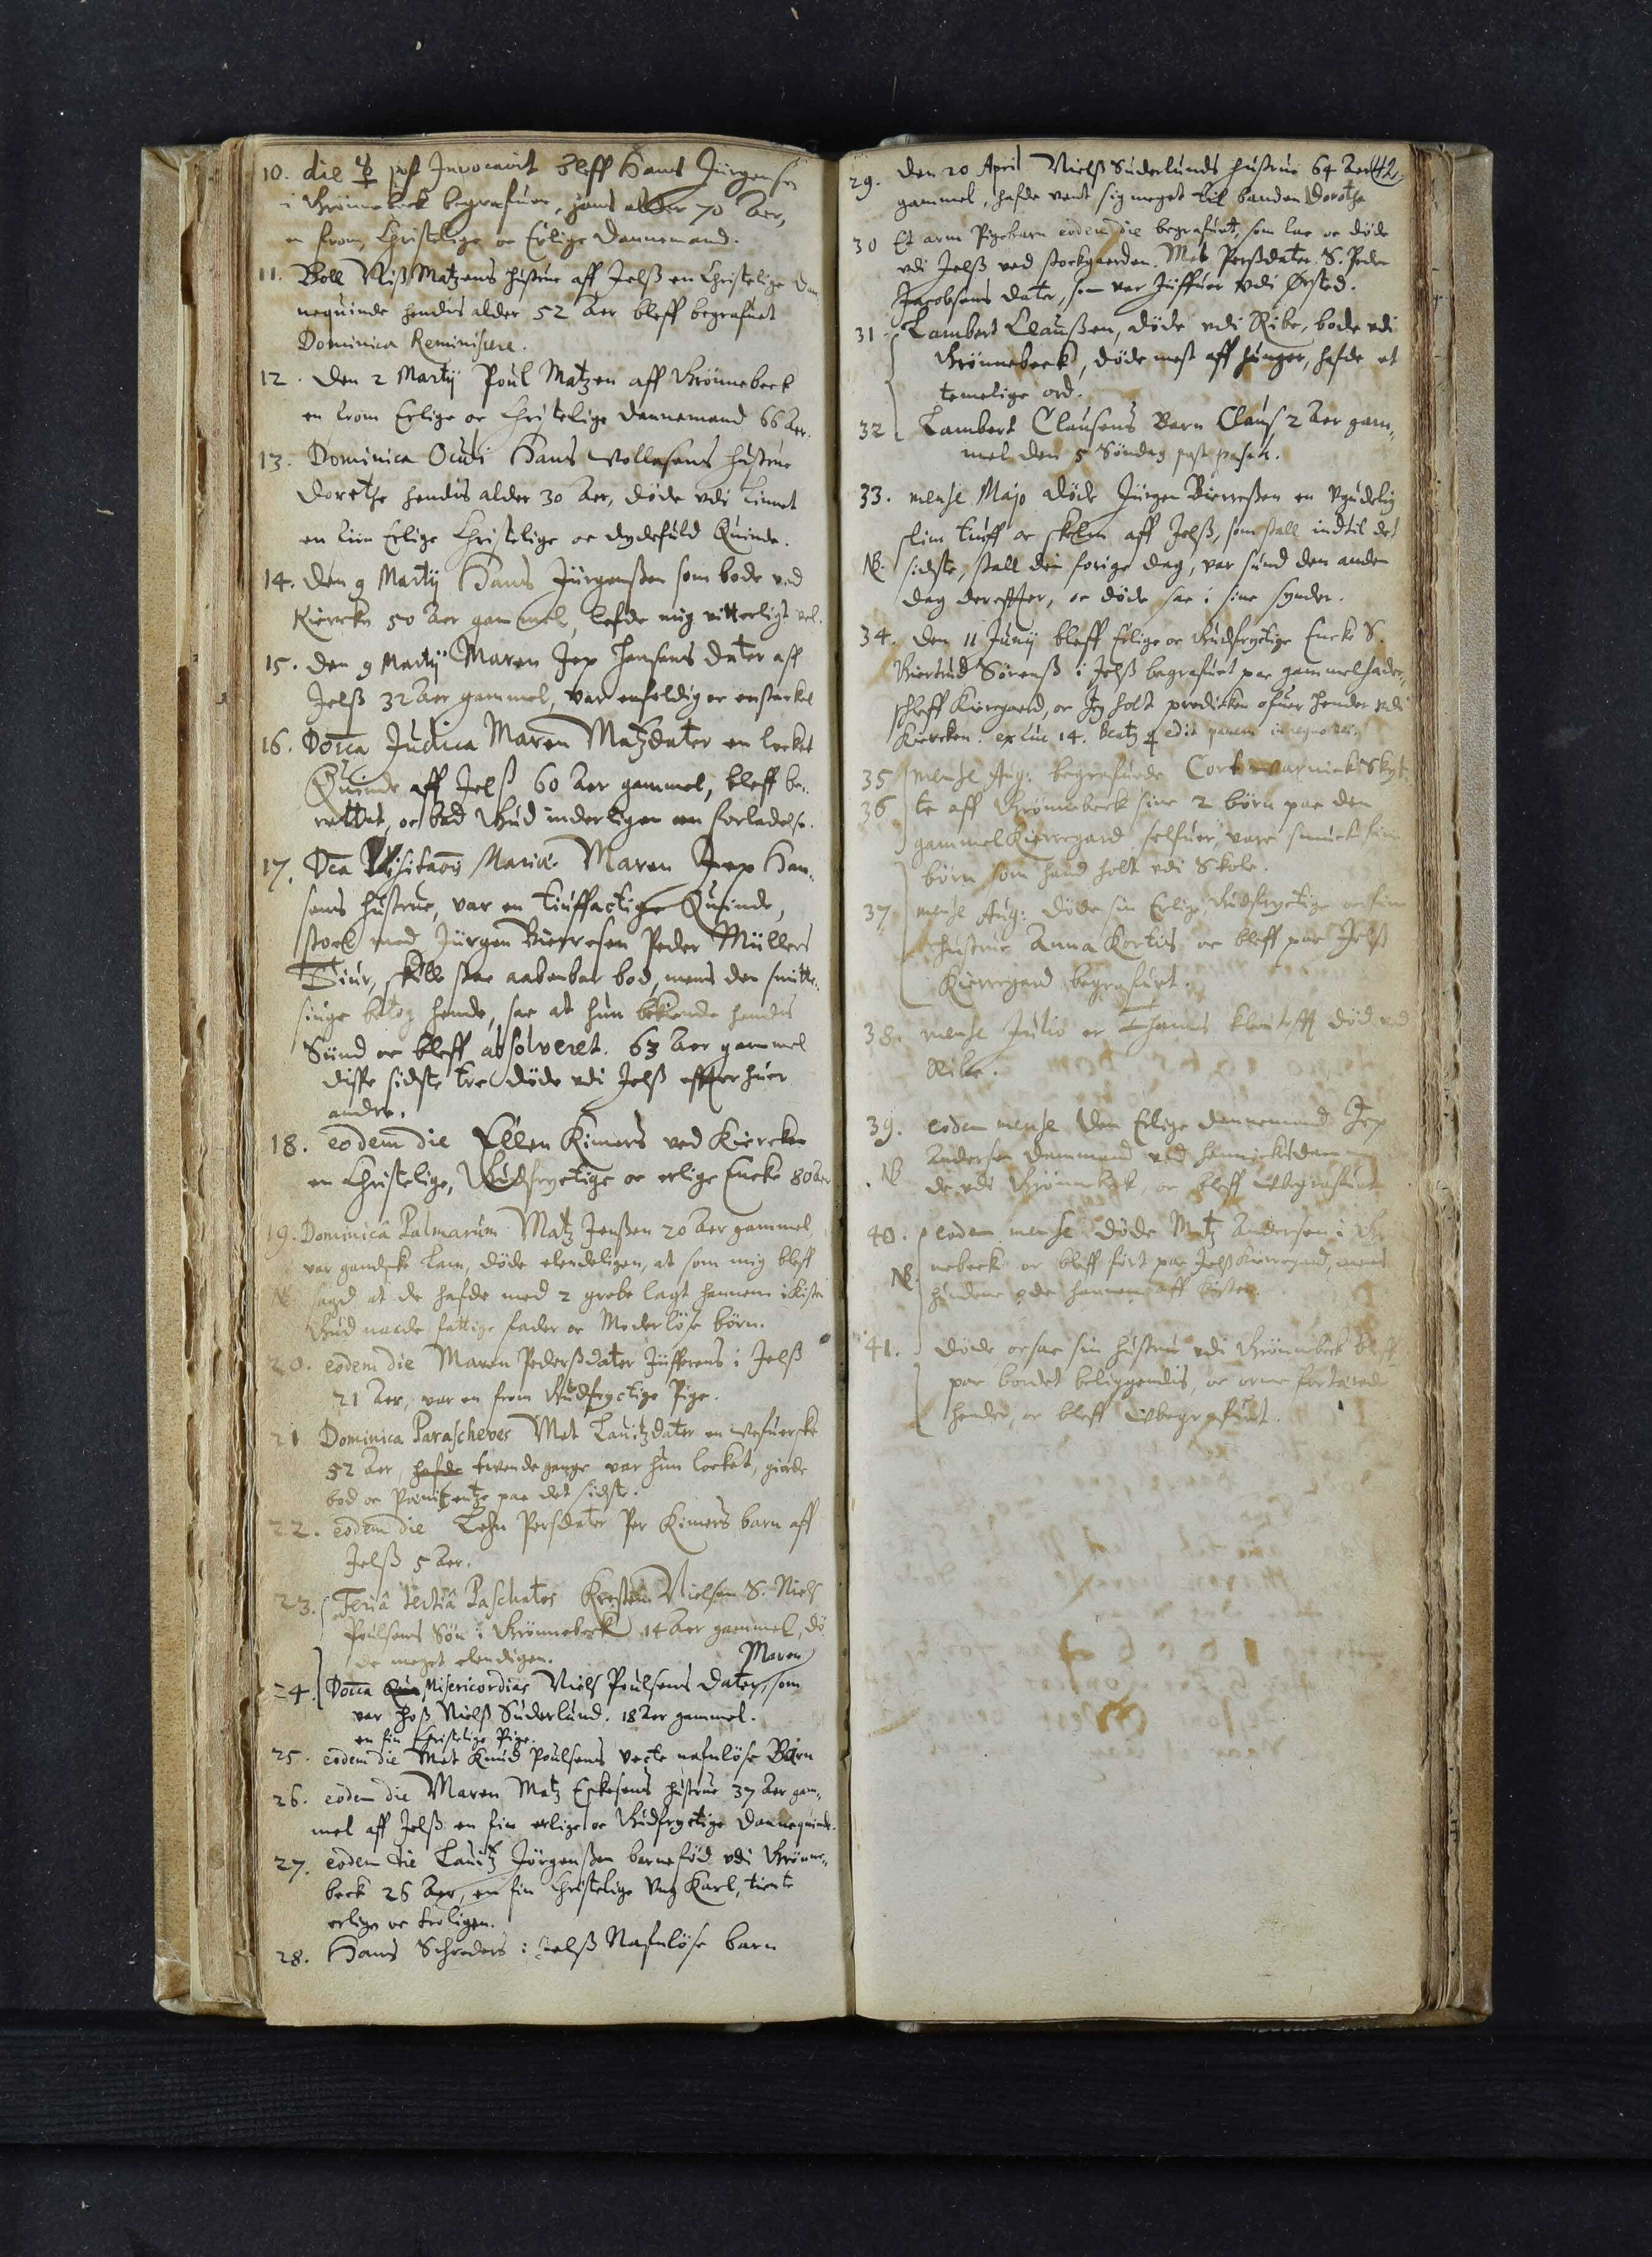10. die ☿ 8 post Invocavit bleff Hans Jurgensen
i Grønnebeck begrafuet, hans alder 70 Aar,
en from Christelige oc Erlige Dannemand.
11. Boll Niss Matzens hustrue aff Jelss en Christelige Dan¬
nequinde hendis alder 52 Aar bleff begrafuet 
Dominica Reminiscere.
12. Den 2 Martijy Poul Matzen aff Grønnebeck
en from Erlige oc Christelige Dannemand 66 Aar.
13. Dominica Oculi Hans Vollesens hustrue
Dorethe hendis alder 30 Aar, døde vdi Linnet
en fiin Erlige Christelige oc dydefuld Quinde.
14. Den 9 Martij Hans Jurgensen som bode ved
Kiercken 50 Aar gammel, lefde mig vitterligt vel.
15. Den 9 Martij Maren Jep Hansens Dater aff
Jelss 32 Aar gammel, var enfoldig oc en stackel.
16. Doica Judica Maren Matzdater en locket
Quinde aff Jels 60 Aar gammel, bleff be¬
rettet, oc bad Gud inderligen om forladelse.
17. Dca Visitaonis Maria. Maren Jep Han¬
sens hustrue, var en tiuffactige Quinde,
stoel med Jurgen Bierresen Peder Mullers
Tiuv, skulle stae aabenbar bod, men den smitte¬
siuge betog hende, sae at hun bekiende hendis
Sund oc bleff absolveret. 63 Aar gammel
Disse sidste tre døde vdi Jelss efter huer
andre.
18. eodem die Ellen Kimers ved Kiercken
en Christelige, Gudfryctige oc erlige Encke 80 Aar
19. Dominica Palarum Matz Jenssen 20 Aar gammel,
var gandske lam, døde elendigen, at som mig bleff
NB sagd at de hafde med 2 grebe lagt hannem i kisten
Gud naede fattige fader oc Moderløse børn.
20. eodem die Maren Pederssdater Jufferens i Jelss
21 Aar, var en from Gudfryctige Pige.
21. Dominica Parascheves Met Lauitzdater en vefuerske
52 Aar, hafde tvende gange var hun locket, giorde
bod oc Psnitentze paa det sidste.
22. eodem die Lehn Persdater Per Kimers barn aff
Jelss 5 Aar.
23. Feria tertia Paschatos Kresten Nielsen S. Niels
Poulsens Søn i Grønnebeck 14 Aar gammel, dø
de meget elendigen.
Maren
24. Docca ## Misericordias Niels Poulsens Dater, som
var hoss Nielss Suderlund. 18 Aar gammel.
en fin Christelige Pige.
25. eodem die Met Knud Poulsens vecte nafnløse Barn
26. eodem die Maren Matz Eskesens hustrue 37 Aar gam¬
mel aff Jelss en fin erlige oc Gudfryctige Dannequinde.
27. eodem die Lauitz Jurgenssen barnefød vdi Grønne¬
beck 26 Aar, en fin Christelige vng Karl, tiente
erlige oc troligen.
28. Hans Schreders i Jels nafnløse barn
42
29. Den 20 April Nielsse Suderlunds hustru 64 Aar
gammel, hafde vent sig noget til banden. Dorotha
30 Et arm Pigevarn eodem die begrafuet, som lae og døde
udi Jelss ved storkgaarden. Met Perssdater S. Peder
Jacobsens Dater, som var Juffuer vdi Ørsted.
31. Lambert Claussen, døde vdi Ribe, bode vdi
Grønnebeck, døde mest aff hunger, hafde et 
temelige ord
32. Lambert Clausens Barn Claus 2 Aar gam¬
mel den 5 Søndag post pasch.
33. mense Majo døde Jurgen Bierressen en Vgudelig
slim tiuff oc skelm aff Jelss, som stall indtil det
NB. sidste, stall den forige Dag, var sund den anden
dag derefter, oc døde sae i sine synder.
34. Den 11 Junij bleff Erlige oc Gudfryctige Encke S.
Giertrud Sørenss i Jelss begrafuet paa gammelhader¬
sleff Kiercgaard, oc Jeg holt predicken ofuer hende vdi
Kiercken. sx luc 14. Beatq## † edia## pasen## in##
35 mense Aug. begrafuede Cort Varnick Skyt
36 te aff Grønnebeck sine 2 børn paa den
gammel Kiercegard selfuer, vare## smuck sine
børn som hand holt vdi Skole.
37 mense Aug. Døde sin Erlige Gudfryctige oc fine
hustrue Anna Kortis oc bleff paa Jelss
Kiercegard begrafuet.
38. mense Julio er Thames Kloutoft død ved
Ribe
39. eodem mense den Erlige Dannemand Jep
NB Andersen D##mand ved Hennicksdam##
de vdi Grønnebeck oc bleff vbegrafuet.
40. eodem mense døde Matz Andersen i
NB. nebeck oc bleff ført paa Jelss Kiercegard, men
hundene ode hannem aff kisten.
41. døde ocsae sin hustrue vdi Grønebeck bleff
pae bordet beliggendis oc ormer fortarede
hende oc bleff vbegrafuet.